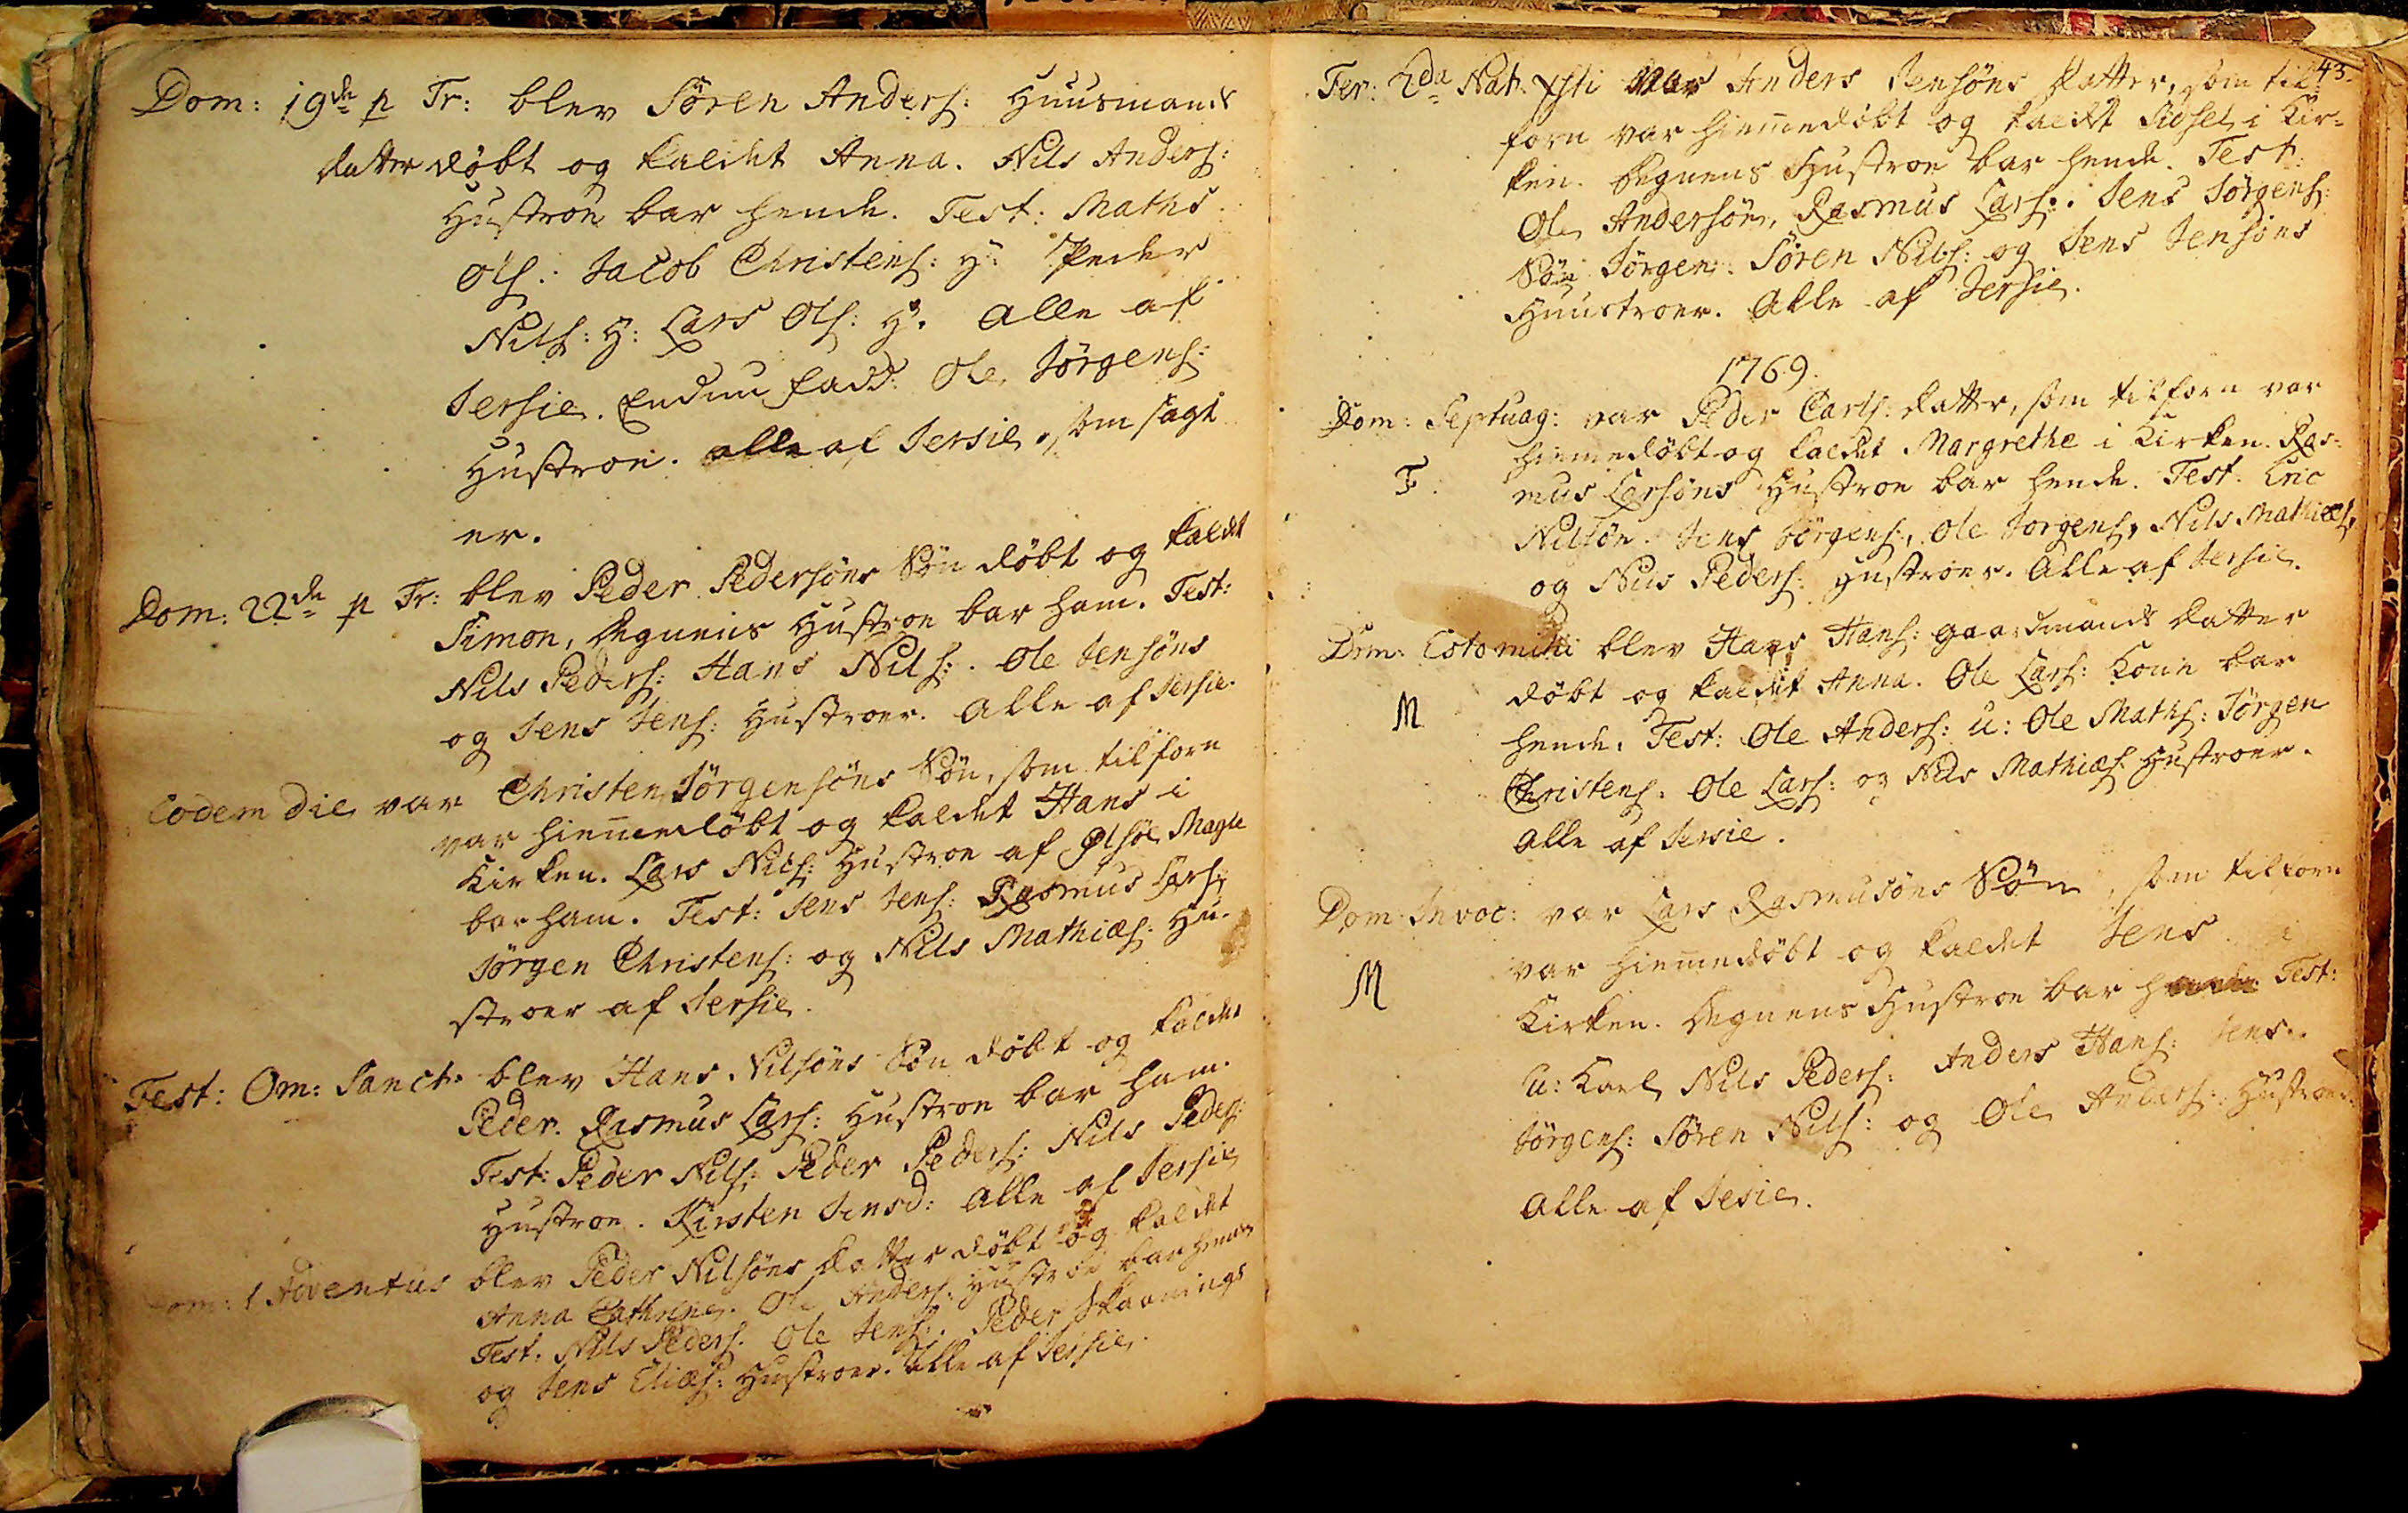Dom: 19de p Tr: blev Søren Anders: Huusmands
Datter døbt og kaldet Anna. Nils Anders:
Hustroe bar Hende. Test: Maths
Ols: Jacob Christens: H: Peder
Nils: H: Lars Ols: H: Alle af
Jersie. Endnu fadd: Ole Jørgens:
Hustroe. Alle af Jersie, som sagt
er.
Dom: 22de p Tr: blev Peder Pedersøns Søn døbt og kaldt
Simon, Degnens Hustroe bar ham. Test:
Nils Peders: Hans Nils: Ole Jensøns
og Jens Jens: Hustroer. Alle af Jersie.
Eodem Die var Christen Jørgensøns Søn, som tilforn
var hiemmedøbt og kaldet Hans i
Kirken. Lars Nils: Hustroe af Ølsøe Magle
bar ham. Test: Jens Jens: Rasmus Lars:
Jørgen Christens: og Nils Mathiæs: Hu¬
stroer af Jersie.
Fest: Om: Sanct: blev Hans Nilsøns Søn døbt og kaldet
Peder. Rasmus Lars: Hustroe bar ham.
Test: Peder Nils: Peder Peders: Nils Peders:
Hustroe. Kirsten Jensd: Alle af Jersie.
Dom: 1. Adventus blev Peder Nilsøns Datter døbt og kaldet
Anna Cathrine. Ole Anders. Hustroe bar hende
Test: Nils Peders. Ole Jens. Peder Skaanings
og Jens Eliæs: Hustroer. Alle af Jersie.
43.
Fer: 2da Nat: Xsti var Anders Jensøns Datter, som til¬
forn var hiemmedøbt og kaldet Sidsel, i Kir¬
ken. Degnens Hustroe bar hende. Test:
Ole Andersøn, Rasmus Lars: Jens Jørgens:
Søn Jørgen. Søren Nils: og Jens Jensøns
Hustroer. Alle af Jersie.
1769.
Dom: Septuag: var Peder Carls: Datter, som tilforn var
F
hiemmedøbt og kaldet Margrethe i Kirken. Ras¬
mus Larsøns Hustroe bar hende. Test: Eric
Nilsøn. Jens Jørgens:, Ole Jørgens: Nils Mathiæs:
og Nils Peders: Hustroer. Alle af Jersie.
Dom: Esto mihi blev Hans Hans: Gaardmands Datter
M
døbt og kaldet Anna. Ole Lars: Kone bar
hende: Test: Ole Anders: u: Ole Maths: Jørgen
Christens: Ole Lars: og Nils Mathiæs: Hustroer.
Alle af Jersie.
Dom: Invoc: var Lars Rasmusøns Søn, som tilforn
M
var hiemmedøbt og kaldet Jens, i
Kirken. Degnens Hustroe bar ham Test:
u: Karl Nils Peders: Anders Hans: Jens 
Jørgens: Søren Nils: og Ole Anders: Hustroer.
Alle af Jersie.
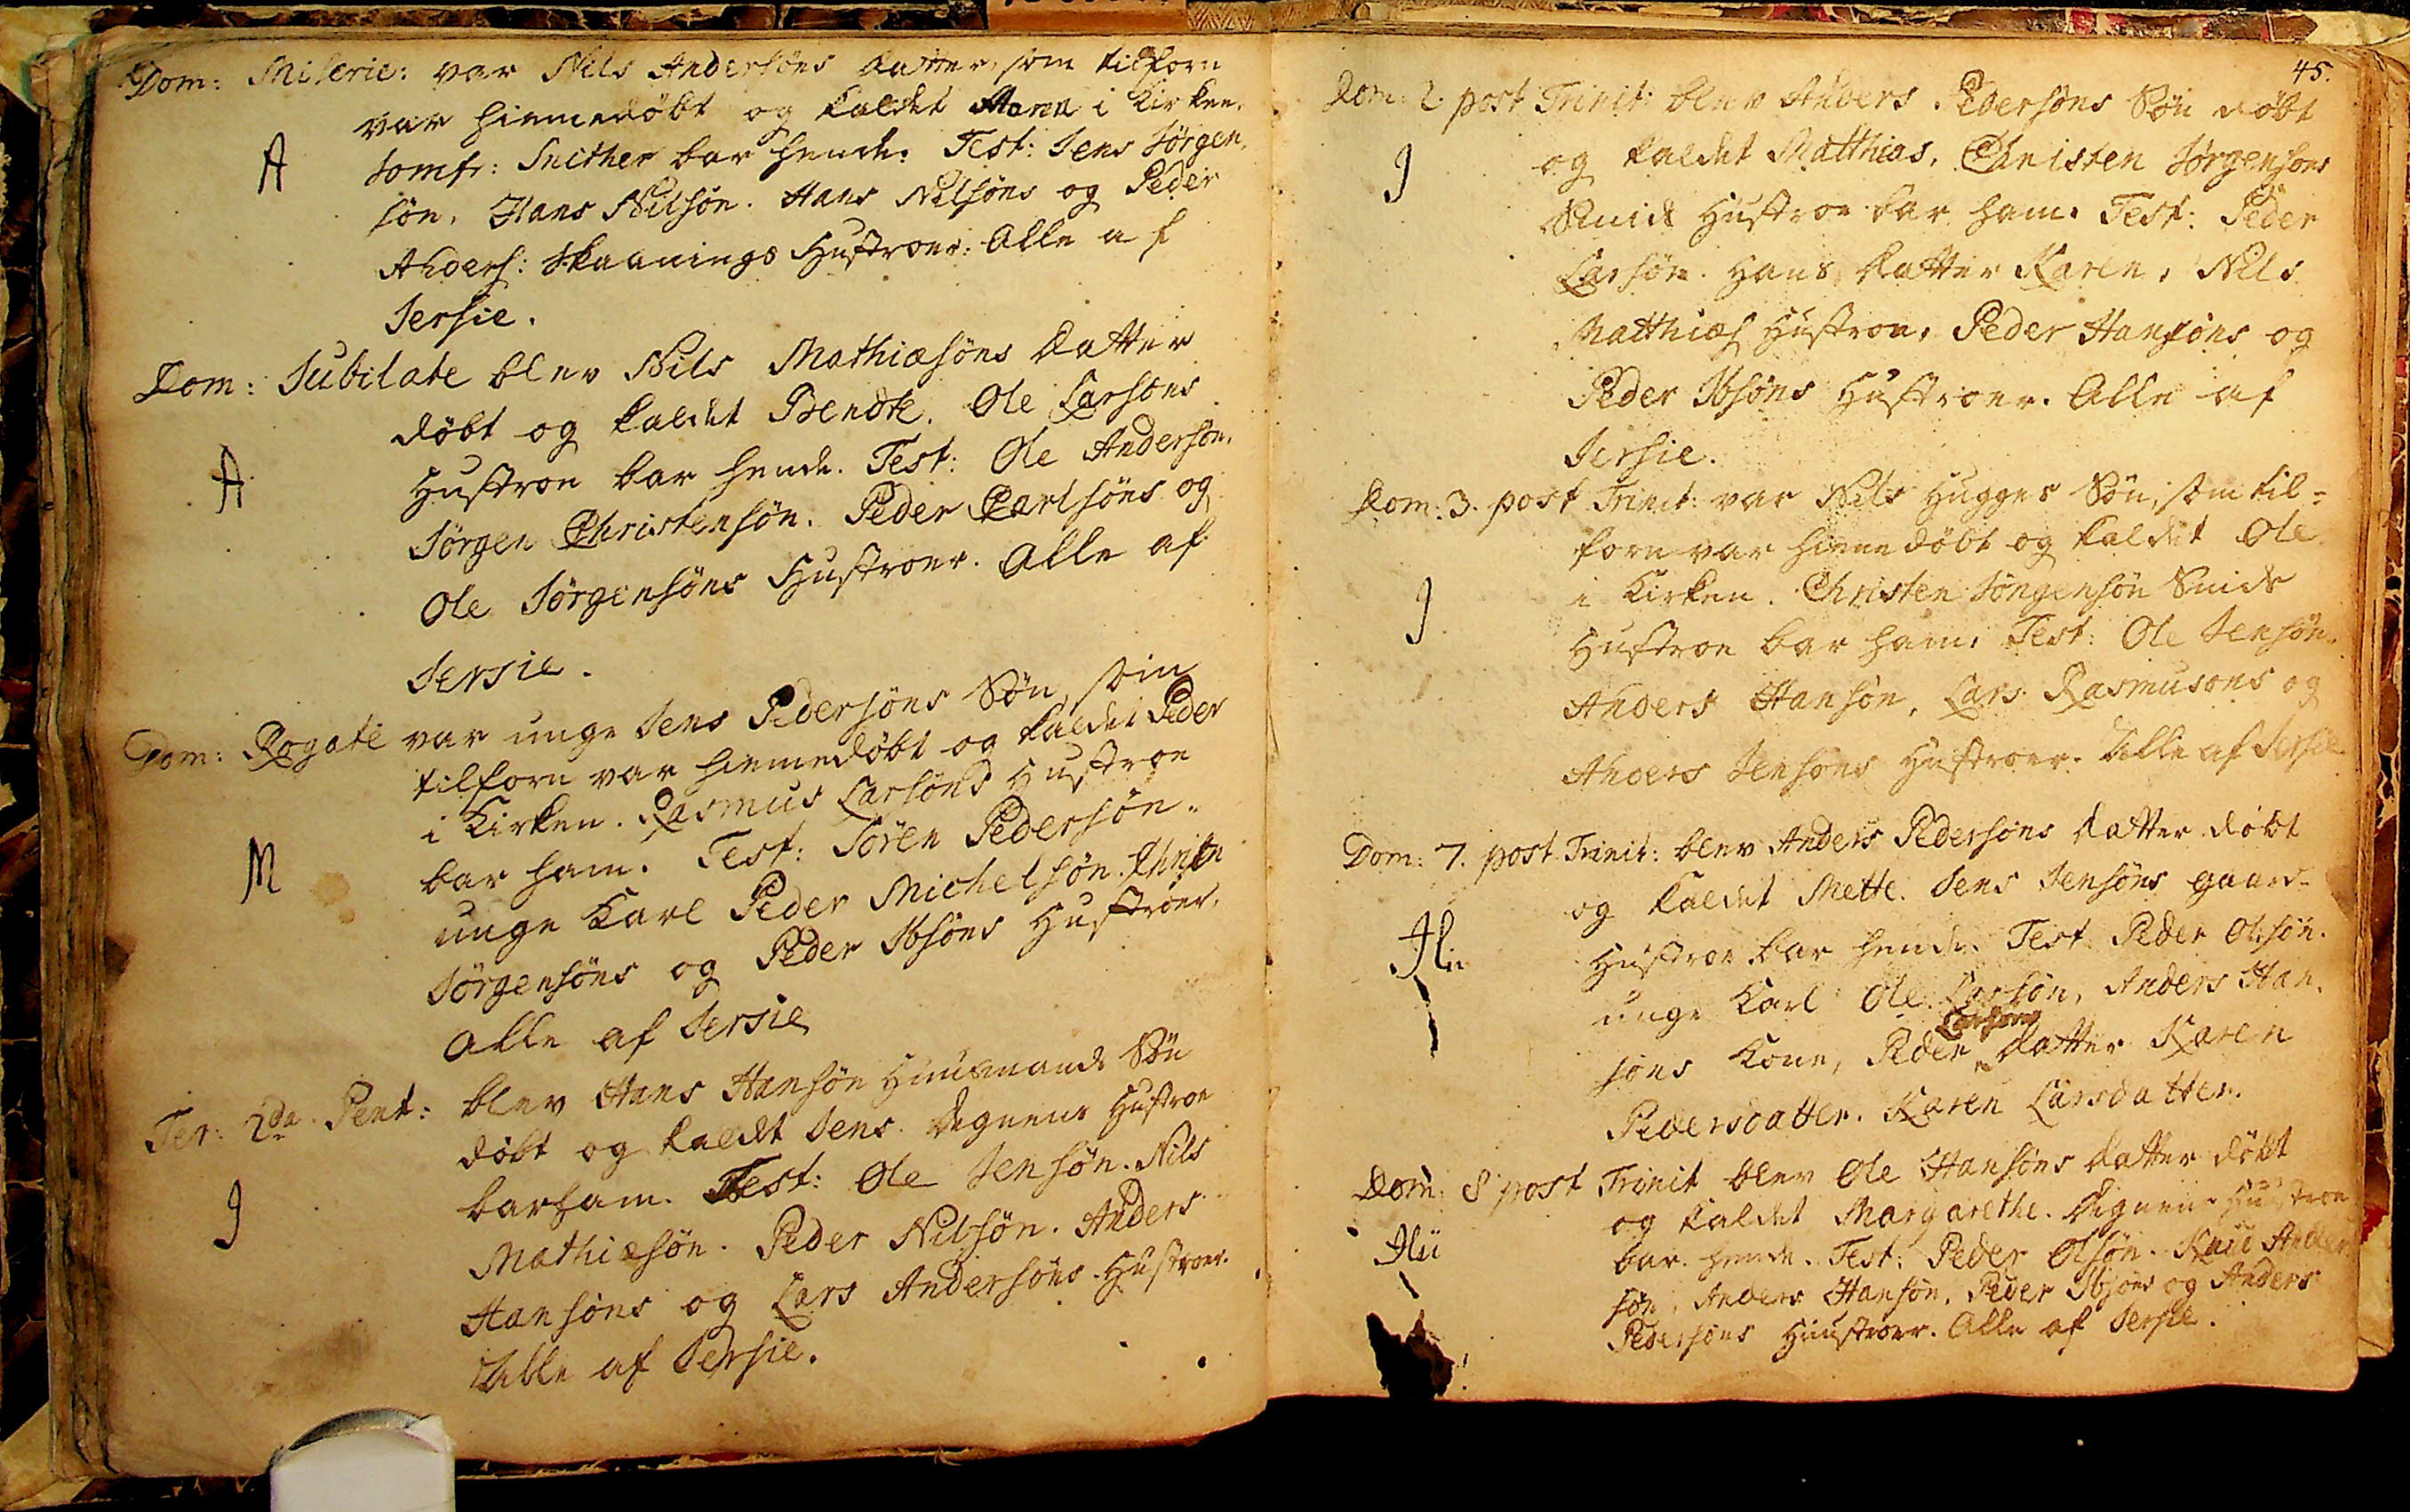Dom: Miseric: var Nils Andersøns Datter, som tilforn
var hiemmedøbt og kaldet Maren i Kirken.
A Jomfr: Snither bar hende: Test: Jens Jørgen¬
søn, Hans Nilsøn. Hans Nilsøns og Peder
Anders: Skaanings Hustroer: Alle af
Jersie.
Dom: Jubilate blev Nils Mathiæsøns Datter
døbt og kaldet Bendte. Ole Larsøns
A  Hustroe bar hende. Test: Ole Andersøn,
Jørgen Christensøn, Peder Carlsøns og
Ole Jørgensøns Hustroer. Alle af
Jersie.
Dom: Rogate var unge Jens Pedersøns Søn, som
tilforn var hiemedøbt og kaldet Peder
i Kirken. Rasmus Larsøns Hustroe
M  bar ham. Test: Søren Pedersøn.
unge Karl Peder Michelsøn. Christn
Jørgensøns og Peder Ibsøns Hustroer.
Alle af Jersie
Fer: 2da Pent: blev Hans Hansøn Huusmands Søn
døbt og kaldt Jens. Degnens Hustroe
J barham. Test: Ole Jensøn. Nils
Mathiæsøn. Peder Nilsøn. Anders
Hansøns og Lars Andersøns Hustroer.
Alle af Jersie
45. 
Dom: 2. post Trinit: blev Anders Pedersøns Søn døbt
og kaldet Matthias. Christen Jørgensøns
J  Smids Hustroe bar ham. Test: Peder
Larsøn Hans Datter Karen, Nils
Matthiæs Hustroe, Peder Hansøns og
Peder Ibsøns Hustroer. Alle af 
Jersie.
Dom: 3. post Trinit: var Nils Huggers Søn, som til¬
forn var hiemmedøbt og kaldet Ole
J  i Kirken. Christen Jørgensøns Smids
Hustroe bar ham. Test: Ole Jensøn,
Anders Hansøn, Lars Rasmussons og
Anders Jensøns Hustroer. Alle af Jersie
Dom: 7. post Trinit blev Anders Pedersøns Datter døbt
og kaldet Mette. Jens Jensøns Gaard:
Hu  Hustroe bar hende. Test: Peder Olsøn.
unge Karl Ole Larsøn, Anders Han¬
Larsøns
søns Kone, Peder Datter Karen
Pedersdatter. Karen Larsdatter.
Dom: 8 post Trinit blev Ole Hansøns Datter døbt
og kaldet Margarethe. Degnens Hustroe
Hu bar hende. Test: Peder Olsøn. Knud Ander
søn, Anders Hansøn, Peder Ibsøns og Anders
Pedersøns Hustroer. Alle af Jersie.